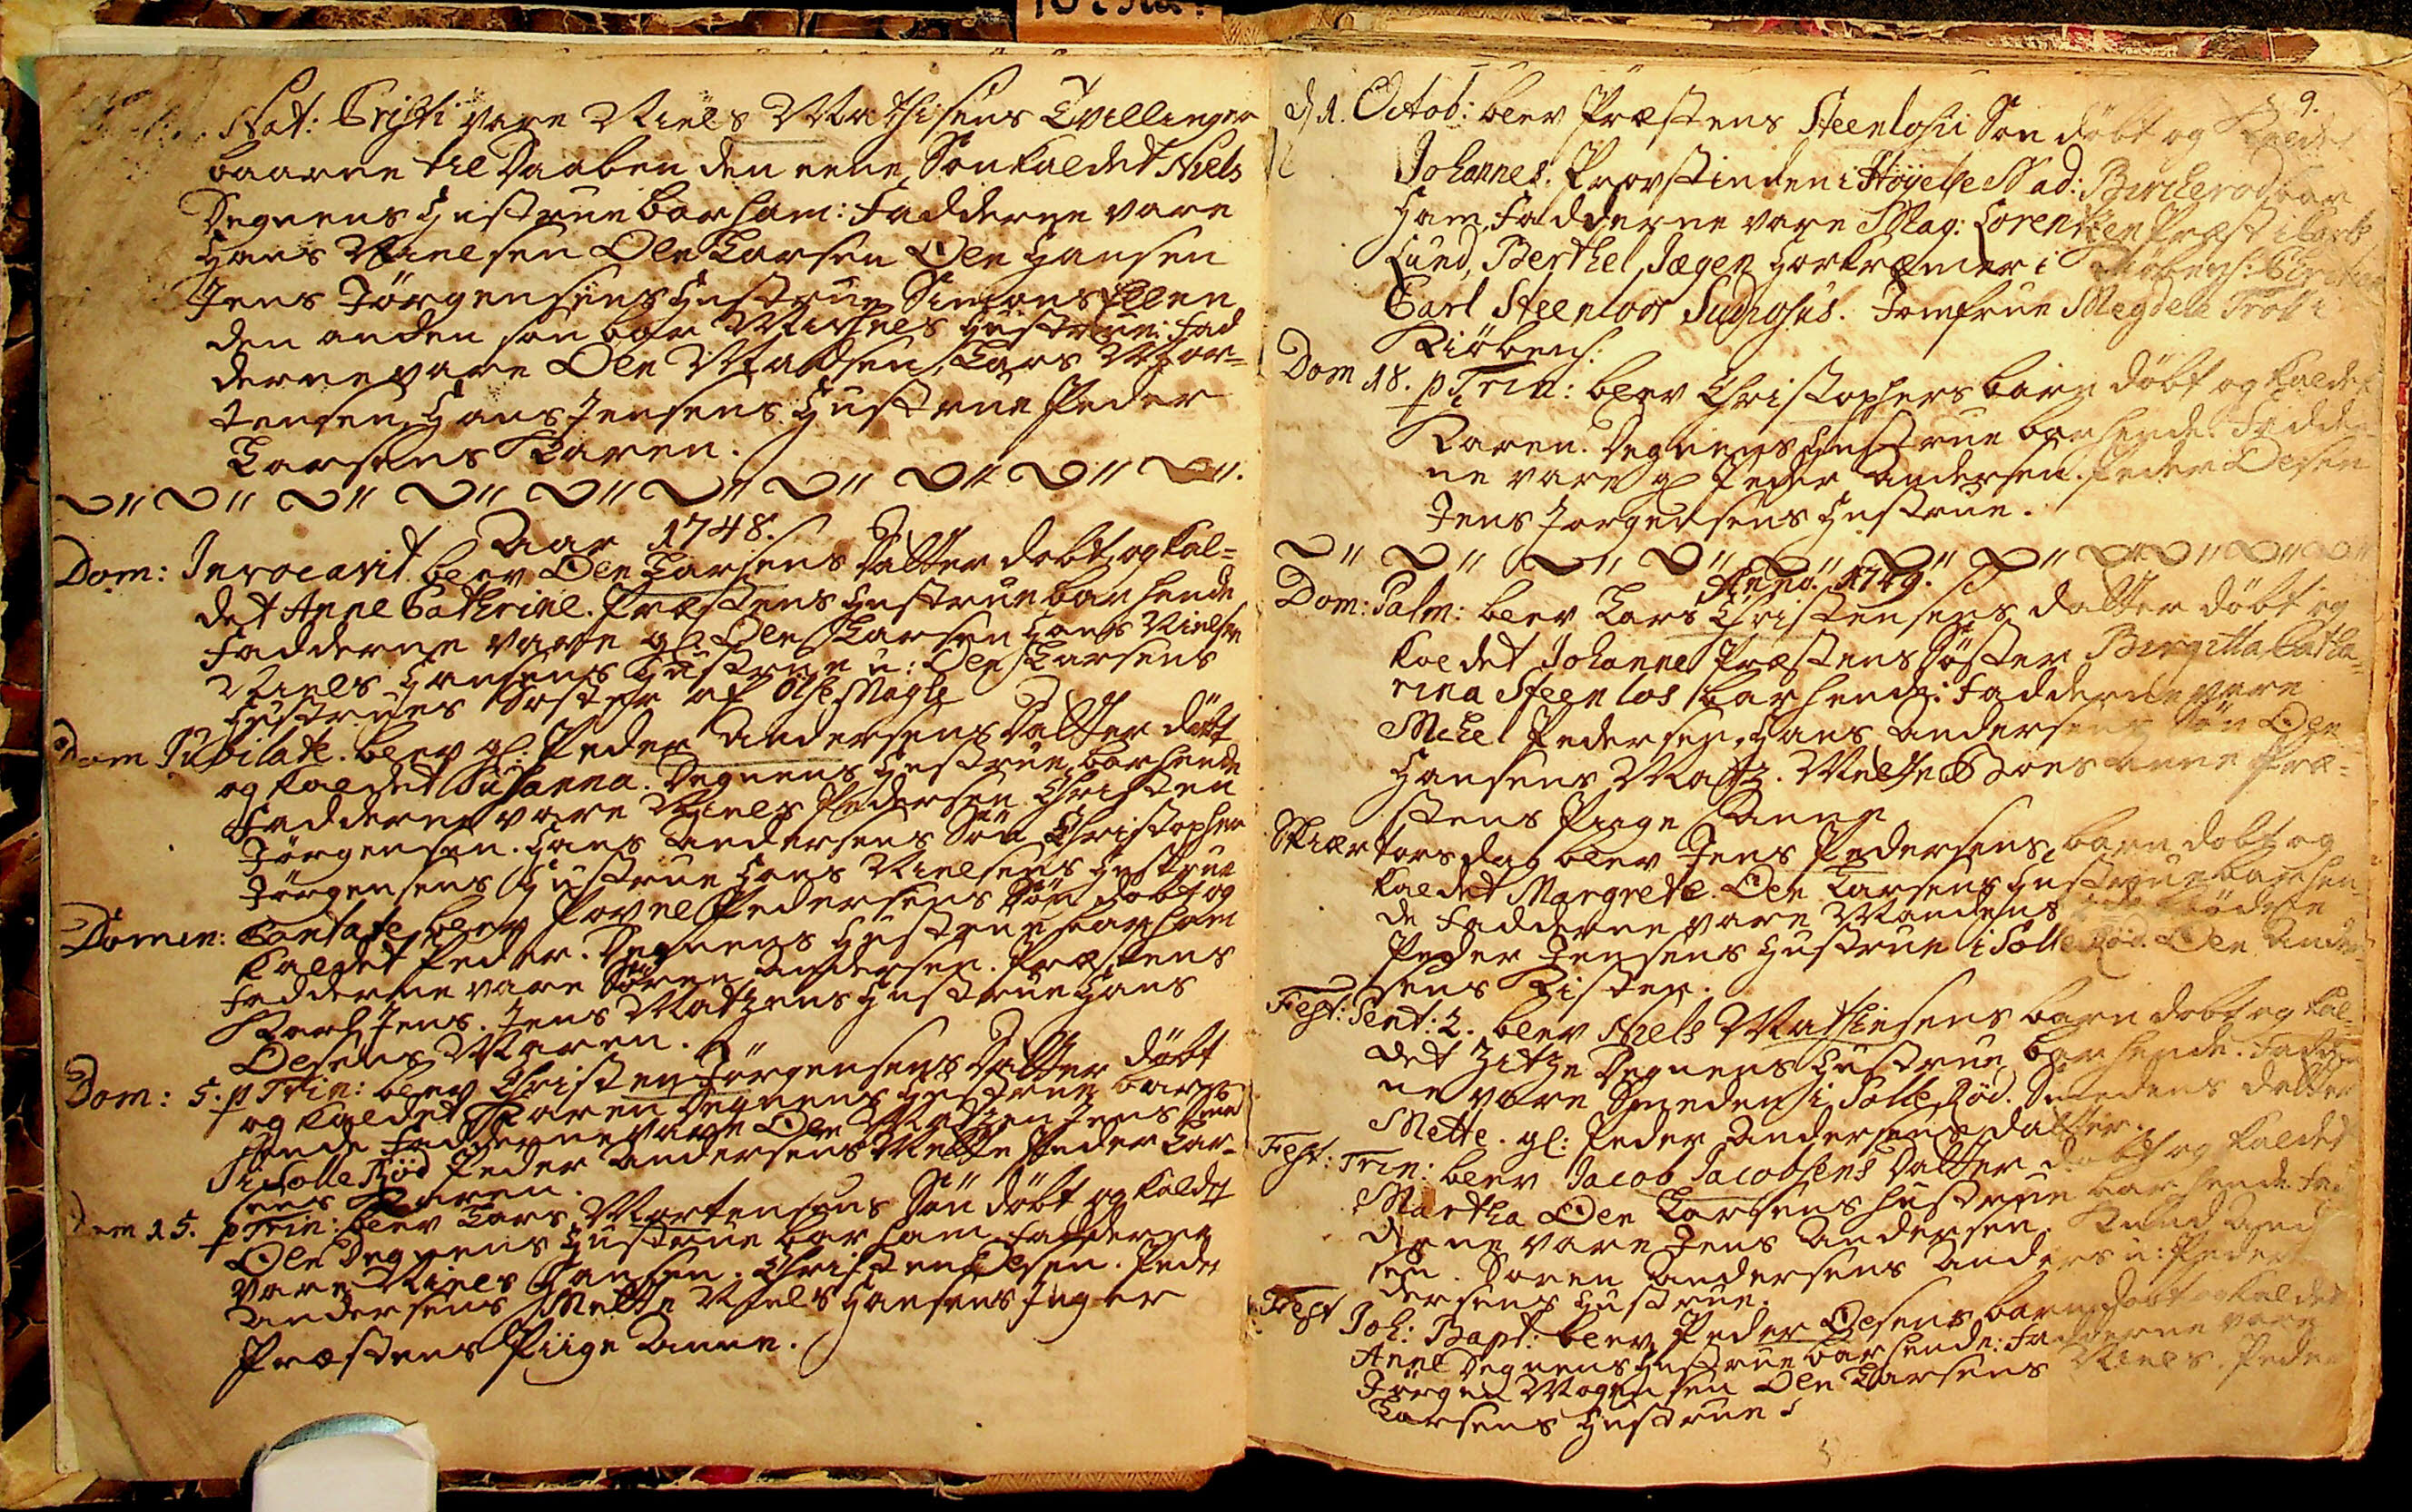Fest 2. Nat: Christi vare Niels Mathisens Zvillinger
baaren til Daaben den eene Søn kaldet Niels
Degnens Hustrue bar ham: Fadderne vare
Hans Nielsen Ole Larsen Ole Hansen
Jens Jørgensens Hustrue Simons Ellen
den anden Søn bar Michels Hustrue: Fad
derne vare Ole Madsen, Lars Mor¬
tensen Hans Jensens Hustrue Peder
Larsens Karen.
Aar 1748.
Dom: Invocavit. blev Ole Larsens Datter døbt og kal¬
det Anne Cathrine. Præstens Hustrue bar hende
Fadderne vare gl: Ole Larsen Hans Nielsen
Niels Hansens Hustrue u: Ole Larsens
Hustrues Søster af Ølse Magle
Dom Jubilate. blev gl: Peder Andersens Datter døbt
og kaldet Susanna. Degnens Hustrue bar hende
Fadderne vare Niels Pedersen, Christen
Jørgensen. Hans Andersens Søn Christopher
Jørgensens Hustrue Hans Nielsens Hustrue
Domin: Cantate blev Povel Pedersens Søn døbt og
kaldet Peder. Degnens Hustrue bar ham
Fadderne vare Søren Andersen. Præstens
Karl Jens. Jens Matzens Hustrue Hans
Olsens Maren.
Dom: 5.p Trin: blev Christen Jørgensens Datter døbt
og kaldet Karen Degnens Hustrue bar##
hende Fadderne vare Ole Matzen Jens Smed
i Solle Rød Peder Andersens Mette Peder Lar¬
sens Karen.
Dom 15.p Trin: blev Lars Mortensens Søn døbt og kaldet
Ole Degnens Hustrue bar ham. Fadderne
vare Niels Hansen. Christen Olsen. Peder
Andersens Mette Niels Hansens Inger
Præstens Piige Anne.
9.
d 1. Octob: blev Præstens Steenlosii Søn døbt og kaldet
Johannes. Provstinden i Høyelse Mad: Bircherod bar
ham, Fadderne vare Mag: Lorentzen Præst i Carls
Lund, Berthel Jægen Hørkræmer i Kiøbenh: Christian
Carl Steenloos Sudofus##. Jomfrue Megdele Trolle i
Kiøbenh:
Dom 18.p Trin: blev Christophers barn døbt og kaldet
Karen. Degnens Hustrue bar hende. Fadder
ne vare gl Peder Andersen. Peder Olsen
Jens Jørgensens hustrue.
Anno 1749.
Dom: Palm: blev Lars Christensens datter døbt og
kaldet Johanne Præstens Søster Birgitta Catha¬
rina Steenlos bar hende: Fadderne vare
Mikel Pedersen. Hans Andersens Søn Ole
Hansens Matz. Mette Boes Anne Præ¬
stens Pige Anne
Skiærtorsdag blev Jens Pedersens barn døbt og
kaldet Margrete. Ole Larsens Hustrue bar hen¬
de Fadderne vare Mandens 2de brødre##
Peder Jensens Hustrue i Solle Rød Ole Ander¬
sens Kirsten.
Fest: Pent: 2. blev Niels Mathiesens barn døbt og kal¬
det Zitze Degnens Hustrue bar hende. Fadder
ne vare Smeden i Solle Rød. Smedens datter
Mette. gl: Peder Andersens datter.
Fest: Trin: blev Jacob Jacobsens Datter døbt og kaldet
Martha Ole Larsens Hustrue bar hende Fad¬
derne vare Jens Andersen Knud And
sen. Søren Andersens Anders u: Peder ##
dersens Hustrue.
Fest Joh: Bapt: blev Peder Olsens barn døbt og kaldet
Anne Degnens Hustrue bar hende: Fadderne vare
Jørgen Mogensen Ole Larsens Niels. Peder
Larsens Hustrue.
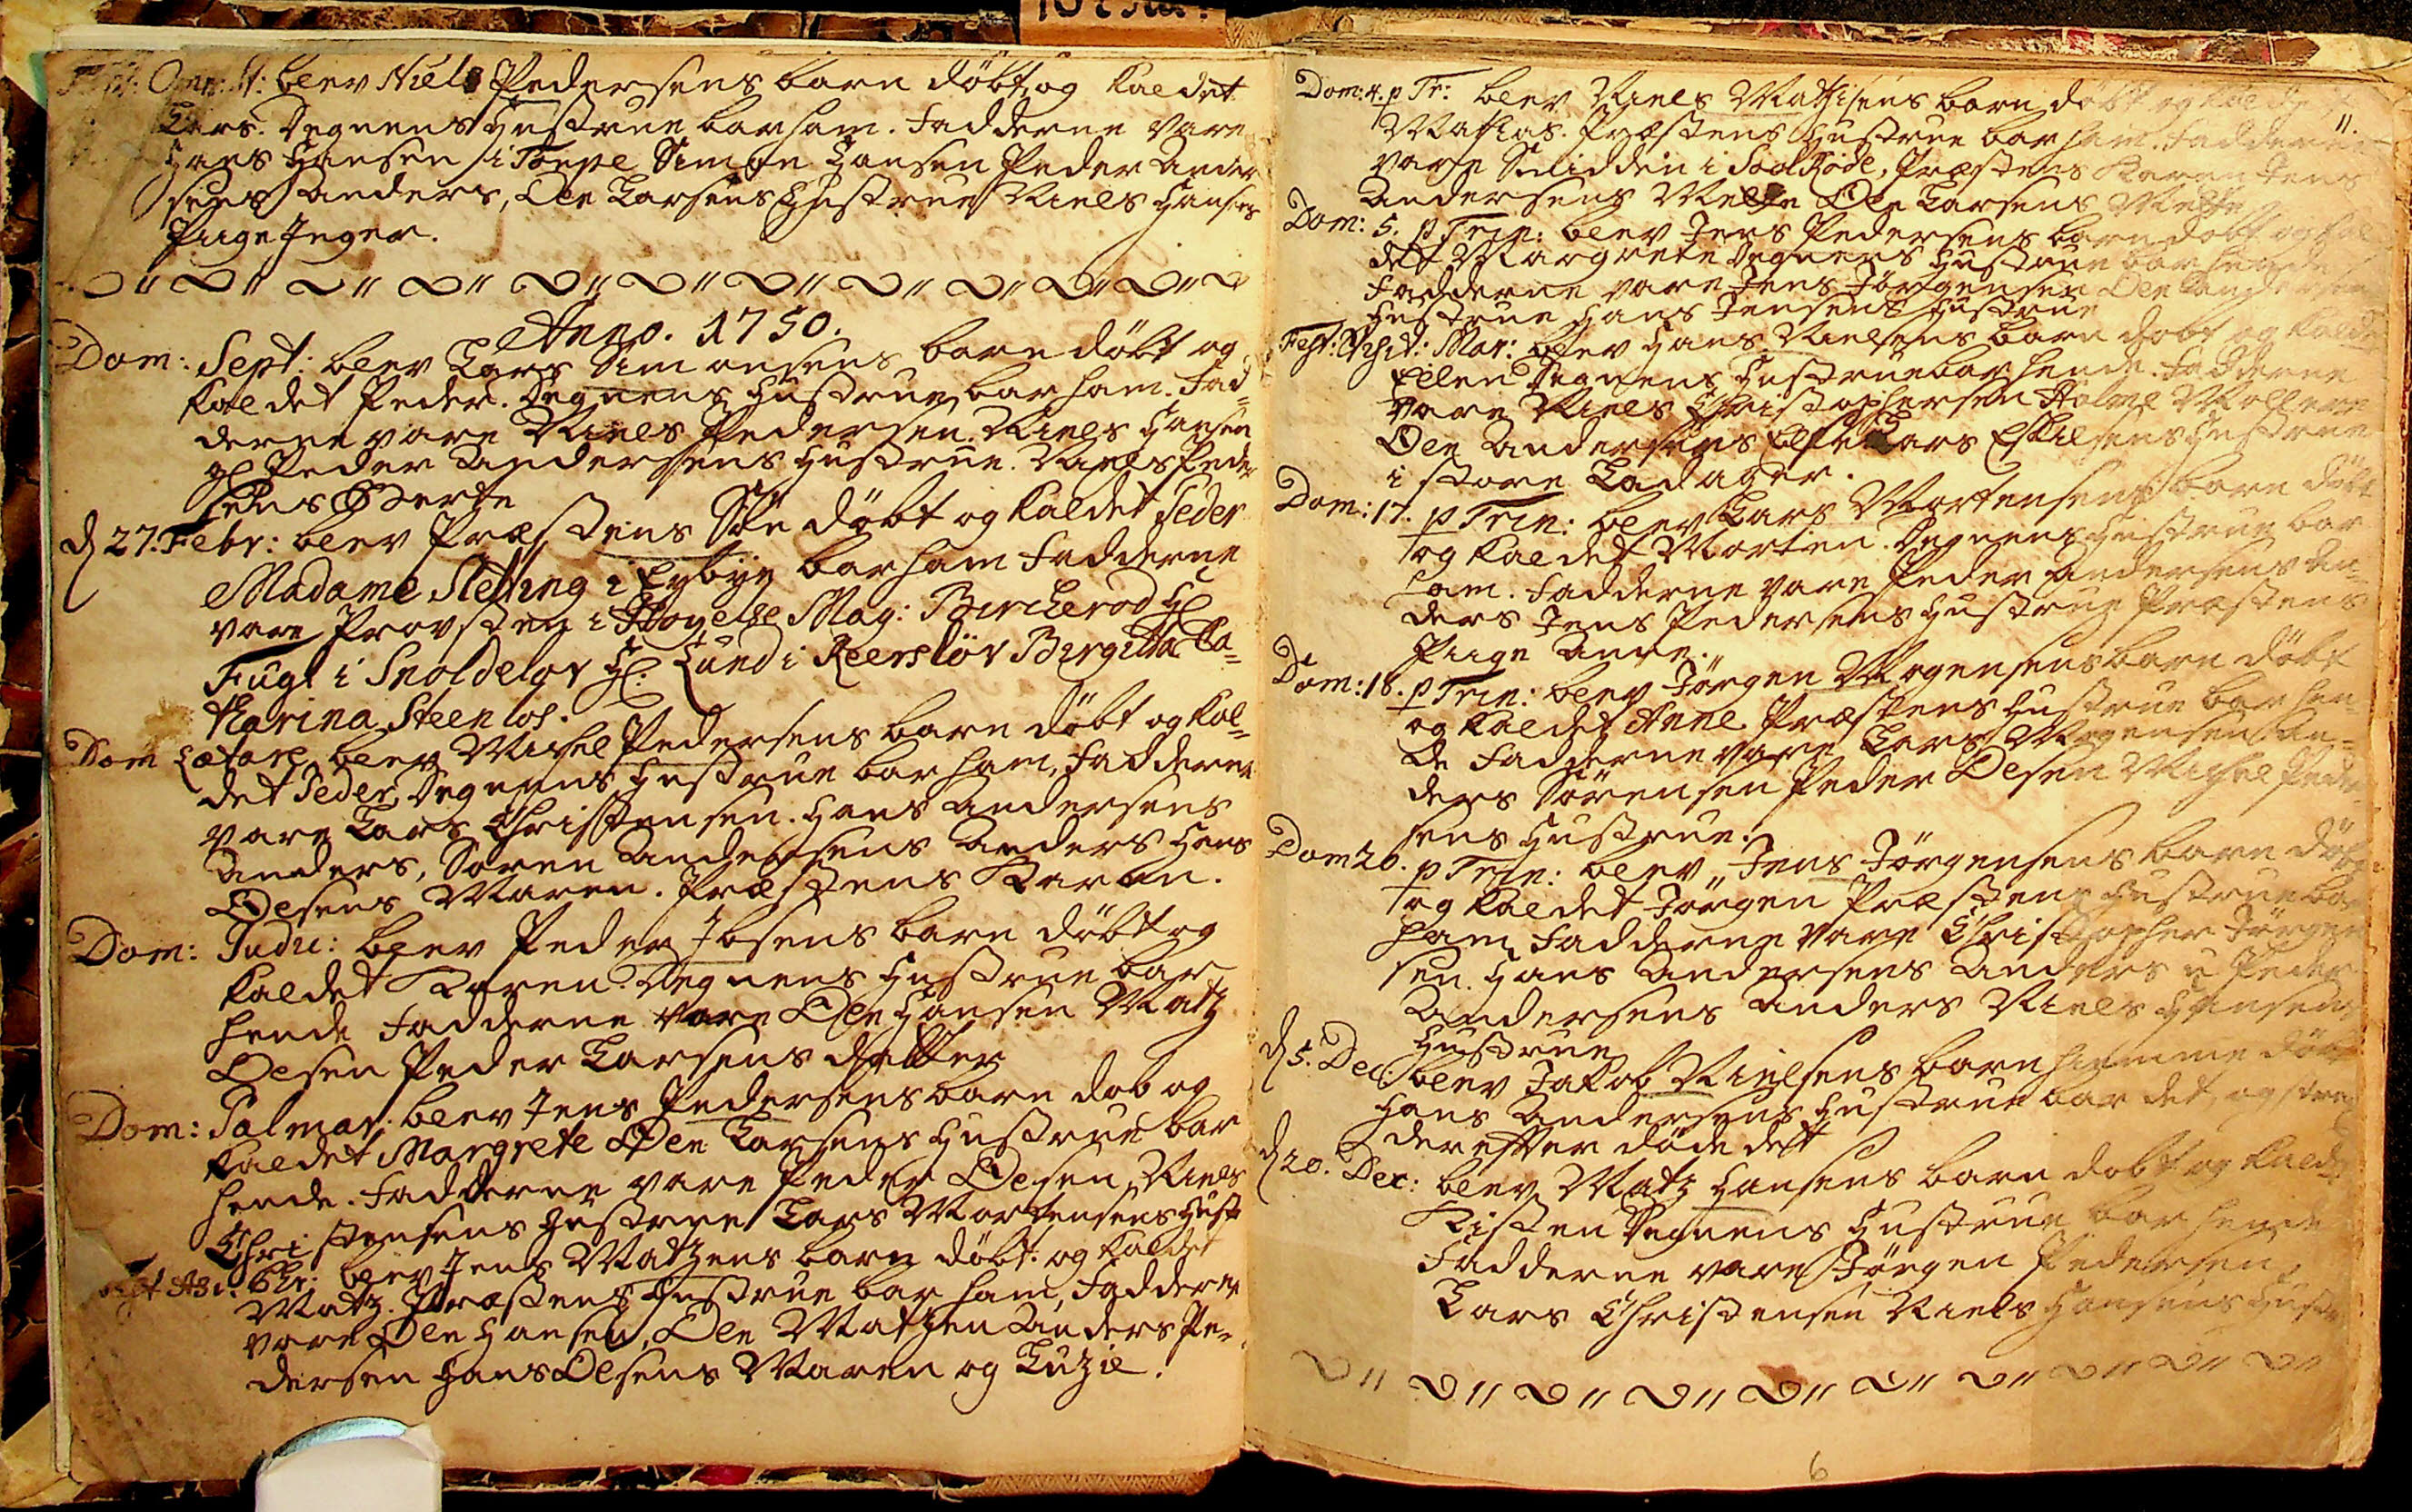Fest: Omn: St: blev Niels Pedersens barn døbt, og kaldet
Lars. Degnens Hustrue bar ham. Fadderne vare
Hans Hansen i Torpe Simon Hansen Peder Ander
sens Anders, Ole Larsens Hustrue Niels Hansens
Pige Inger.
Anno. 1750.
Dom: Sept: blev Lars Simonsens barn døbt og
kaldet Peder. Degnens Hustrue bar ham. Fad¬
derne vare Niels Pedersen. Niels Hansen
gl Peder Andersens Hustrue. Niels Peder¬
sens Berte
d 27. Febr: blev Præstens Søn døbt og kaldet Peder
Madame Sletting i Eybye bar ham Fadderne
vare Provsten i Høyelse Mag: Bircherod Hr
Fugl i Snoldelov Hr: Lund i Reersløv Birgitta Ca¬
tharina Steenlos.
Dom Lætare blev Michel Pedersens barn døbt og kal¬
det Peder, Degnens Hustrue bar ham, Fadderne
vare Lars Christensen. Hans Andersens
Anders, Søren Andersens Anders Hans
Olsens Maren. Præstens Karen.
Dom: Judic: blev Peder Ibsens barn døbt og
kaldet Karen. Degnens Hustrue bar
hende Fadderne vare Ole Hansen Matz
Olsen Peder Larsens datter
Dom: Palmar. blev Jens Pedersens barn dob og
kaldet Margrete Ole Larsens Hustrue bar
hende. Fadderne vare Peder Olsen, Niels
Christensens Hustrue Lars Mortensens Hust
Fest Asc. Chr: blev Jens Matzens barn døbt og kaldet
Matz. Præstens Hustrue bar ham, Fadderne
vare Ole Hansen, Ole Matzen Anders Pe¬
dersen Hans Olsens Maren og Luzie.
11.
Dom: 4. p Tr: blev Niels Mathisens barn døbt og kaldet
Mathias. Præstens Hustrue bar ham. Fadderne
vare Smidden i Sool Røde, Præstens Karen Jens
Andersens Mette Ole Larsens Mehte
Dom: 5. p Trin: blev Jens Pedersens barn døbt og kal
det Margrete Degnens Hustrue bar hende
Fadderne vare Jens Jørgensen Ole Andersens
Hustrue Hans Jensens Hustrue
Fest: Visit: Mar: blev Hans Nielsens barn døbt og kaldet
Ellen Degnens Hustrue bar hende. Fadderne
vare Niels Christophersen Holme Mølleren
Ole Andersens Ellen Lars Eskilsens Hustrue
i Store Ladager.
Dom: 17.p Trin: blev Lars Mortensens barn døbt
og kaldet Morten. Degnens Hustrue bar                   
ham. Fadderne vare Peder Andersens An¬
ders Jens Pedersens Hustrue Præstens
Piige Anne.
Dom: 18.p Trin: blev Jørgen Mogensens barn døbt
og kaldet Anne. Præstens Hustrue bar hen¬
de Fadderne vare Lars Mogensen An¬
ders Sørensen Peder Olsen Michel Peder¬
sens Hustrue.
Dom: 20.p Trin: blev Jens Jørgensens barn døbt
og kaldet Jørgen Præstens Hustrue bar
ham Fadderne vare Christopher Jørgen
sen. Hans Andersens Anders u Peder
Andersens Anders Niels Hansens
Hustrue.
d 5.Dec: blev Jakob Nielsens barn hiemme døbt
Hans Andersens Hustrue bar det og stun
den efter døde det
d 20. Dec: blev Matz Hansens barn døbt og kaldet
Kirsten Degnens Hustrue bar hende
Fadderne vare Jørgen Pedersen
Lars Christensen Niels Hansens Hustr